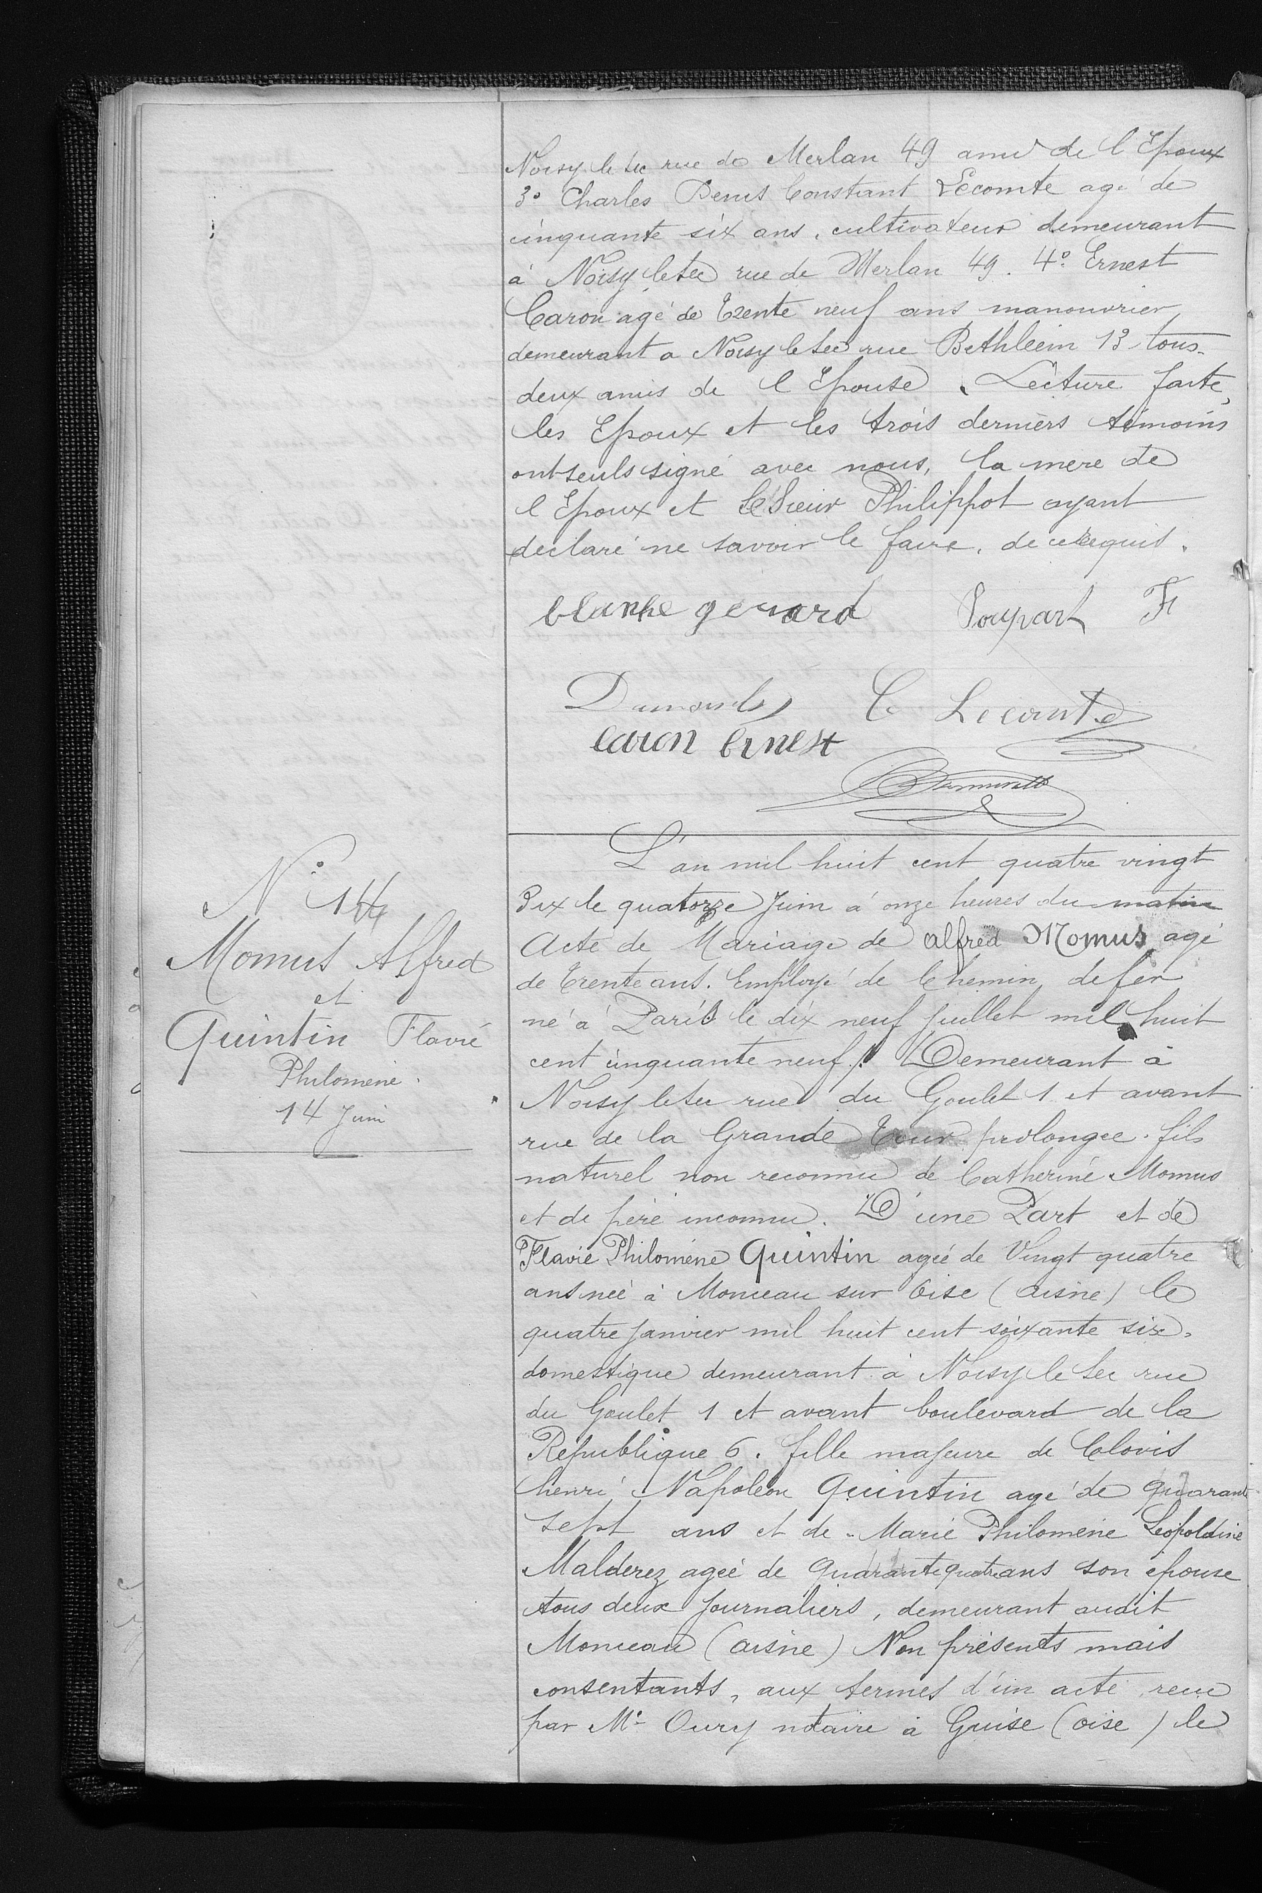Noisy le sec rue de Merlan 49 amis de l'Epoux
3° Charles Denis Constant Lecomte agé de
cinquante six ans, cultivateur demeurant
à Noisy le sec rue de Merlan 49. 4° Ernest
Caron âgé de trente neuf ans manouvrier
demeurant a Noisy le sec rue Bethleein 13, tous
deux amis de l'Epouse Lecture faite,
les Epoux et les trois derniers témoins
ont seuls signé avec nous, la mere de
l Epoux et le Sieur Philippot ayant
declaré ne savoir le faire,de ce requis.
N°144
Momus Alfred
et
Quintin Flavie
Philomene
14 Juin
L'an mil huit cent quatre vingt
dix le quatorze Juin à onze heures du matin
Acte de Mariage de Alfred Momus agé
de Trente ans. Employé de chemin de fer
né à Paris le dix neuf juillet mil huit
cent cinquante neuf Demeurant à
Noisy le sec rue du Goulet 1 et avant
rue de la Grande Tout prolongee fils
naturel non reconnu de Catherine Momus
et de père inconnu. D'une Part et de
Flavie Philomène Quintin agée de vingt quatre
ans née à Monceau sur Oise (Aisne) le
quatre janvier mil huit cent soixante six,
domestique demeurant à Noisy le Sec rue
du Goulet 1 et avant boulevard de la
République 6. fille majeure de Clovis
henri Napoleon Quintin agé de quarante
sept ans et de Marie Philomene Leopoldine
Malderez agée de quarante quatre ans son épouse
tous deux journaliers, demeurant audit
Monceau (Aisne) Non présents mais
consentants, aux termes d'un acte reçu
par Me Oury notaire à Guise (oise) le
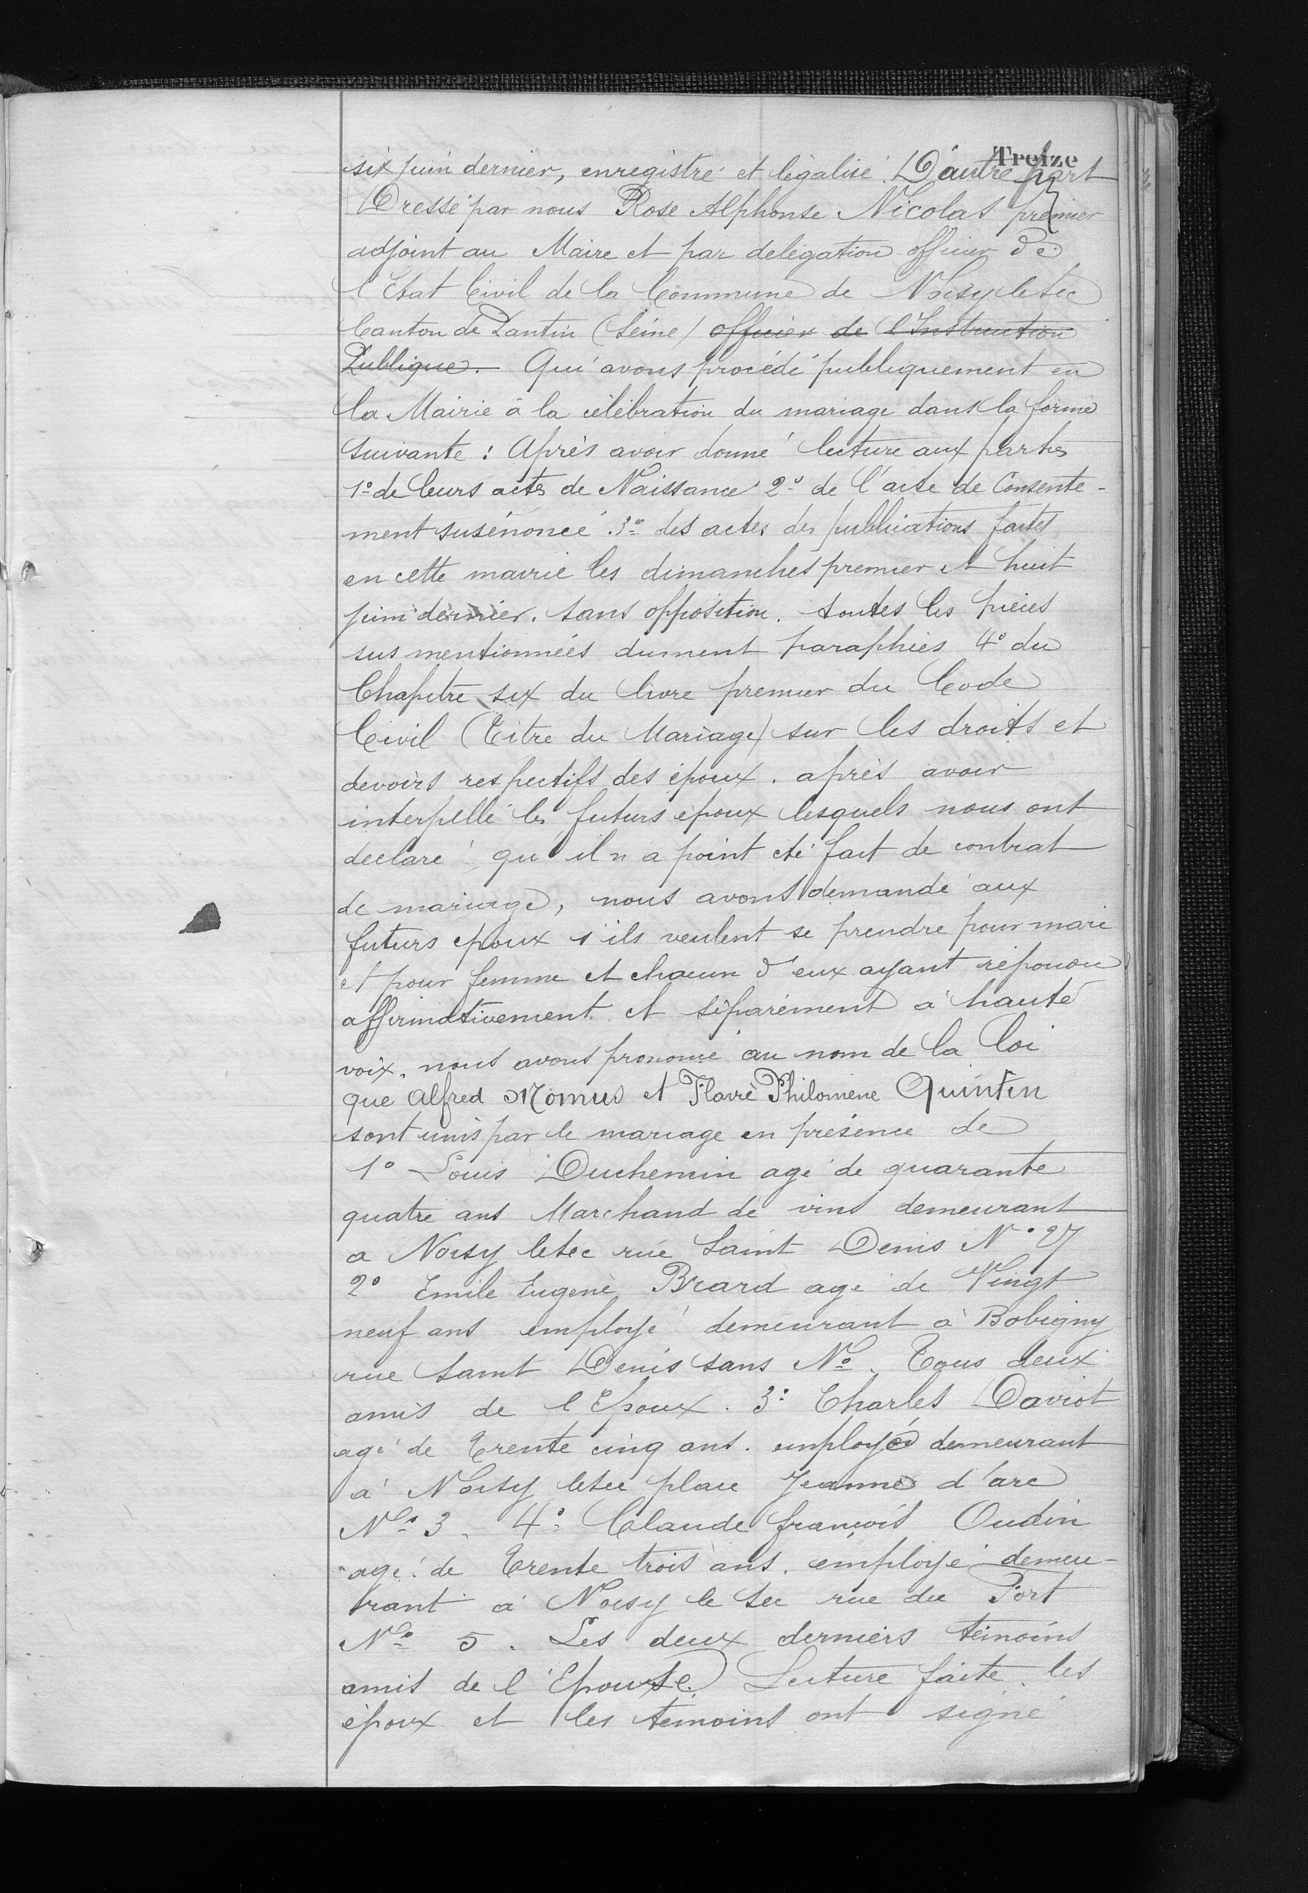six juin dernier, enregistré et légalisé. D'autre part
Dressé par nous Rose Alphonse Nicolas premier
adjoint au Maire et par délégation officier de
l'Etat Civil de la Commune de Noisy le Sec
Canton de Pantin (Seine) officier de l'Instruction
Publique Qui avons procédé publiquement en
la Mairie à la célébration du mariage dans la forme
suivante: après avoir donné lecture aux parties
1°-de leurs actes de Naissance 2°-de l'acte de consente-
ment susénoncé. 3°: des actes des publications faites
en cette mairie les dimanches premier et huit
juin dernier, sans opposition, toutes les pièces
sus mentionnées dument paraphées 4° du
Chapitre six du livre premier du Code
Civil (Titre du Mariage) sur les droits et
devoirs respectifs des époux. après avoir
interpellé les futurs époux lesquels nous ont
declaré qu'il n a point été fait de contrat
de mariage, nous avons demandé aux
futurs epoux s'ils veulent se prendre pour mari
et pour femme et chacun d'eux ayant répondu
affirmativement et séparément à haute
voix, nous avons prononcé au nom de la loi
que Alfred Momus et Flavie Philomène Quintin
sont unis par le mariage en présence de
1° Louis Duchemin agé de quarante
quatre ans Marchand de vins demeurant
a Noisy le sec rue Saint Denis N°27
2° Emile Eugene Brard agé de Vingt
neuf ans employé demeurant à Bobigny
rue Saint Denis sans N° Tous deux
amis de l Epoux. 3° Charles Daviot
agé de trente cinq ans, employé demeurant
à Noisy le sec place Jeanne d'arc
N°3, 4°-Lalande françois Oudin
agé de trente trois ans, employé demeur-
rant à Noisy le sec rue du Fort
N° 5 Les deux derniers témoins
amis de l'Epouse Lecture faite, les
époux et les témoins ont signé